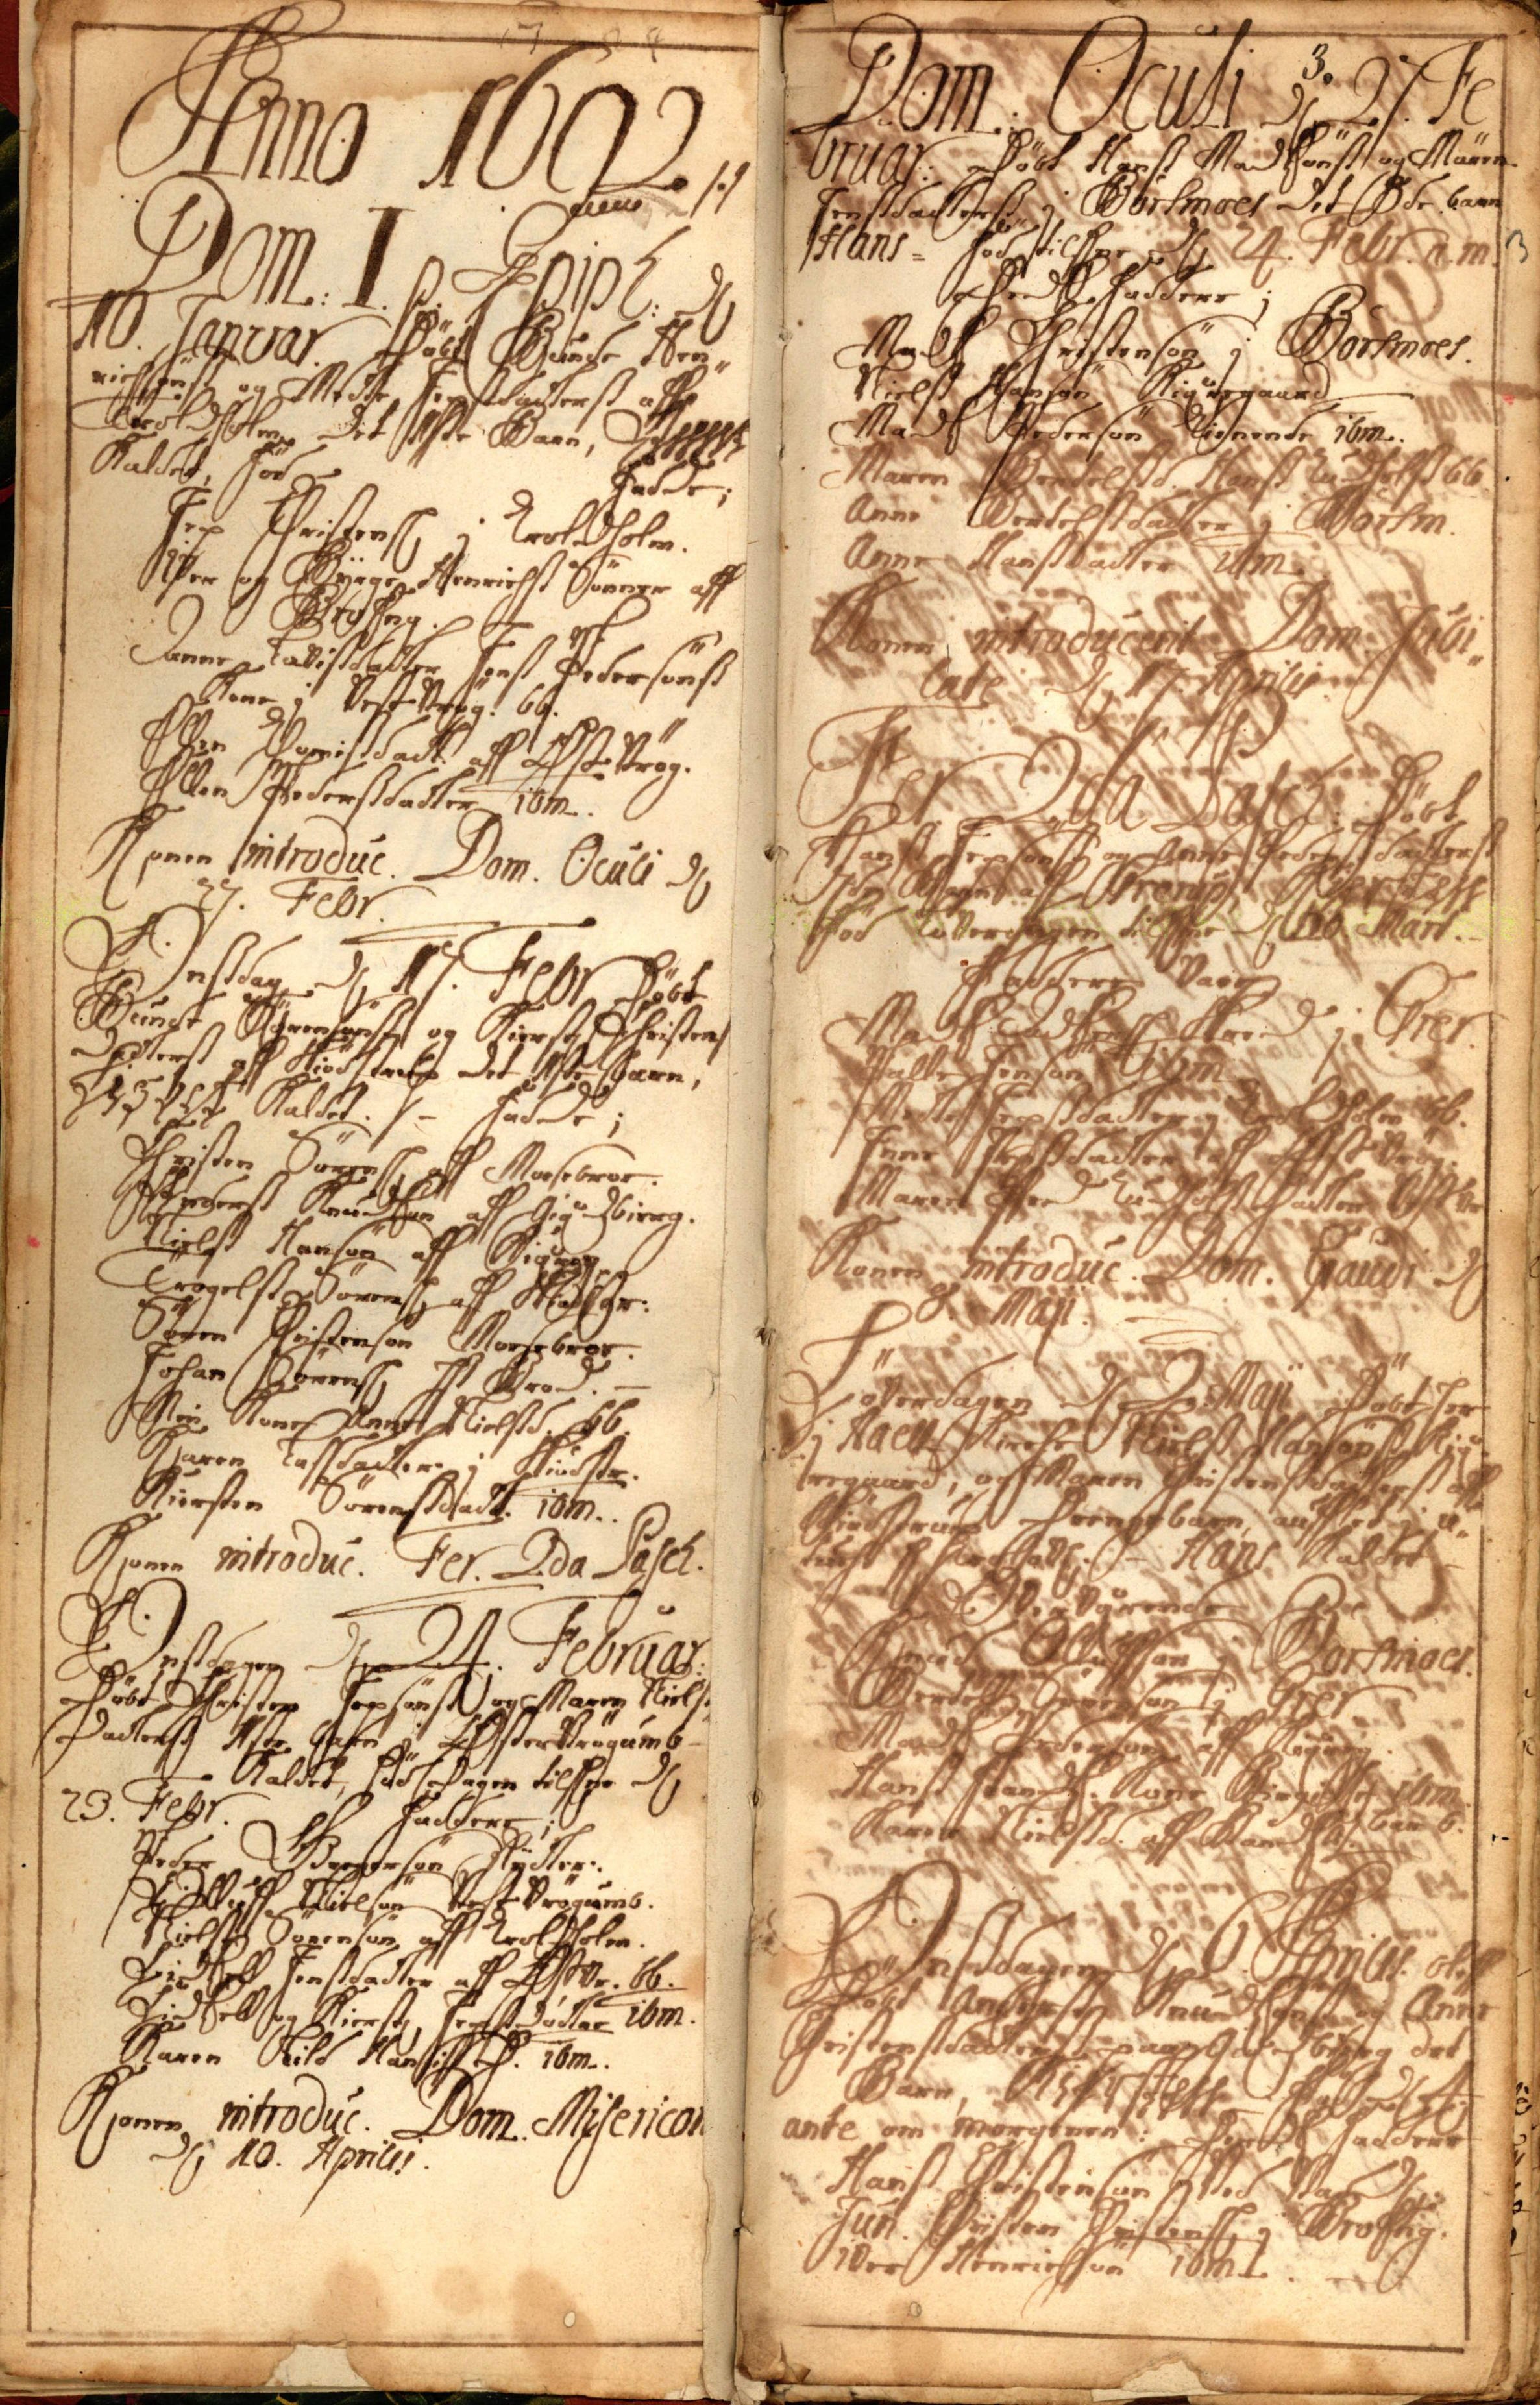Anno 1692
11
Dom:1.p.Epiph:. d
10. Januar. Døbt Bunde Hen¬
richsøn og Mette Jepsdatters aff
Troldholm det 1ste Barn, Anne
kaldet, fød
Fadder
Jep Christens j Troldholm.
Iver og Byrge Henrichs Sønner aff
BroEng.
Anne Lavisdatter Jens Pedersøns
Kone j Vester Vrøg. bb.
Ellen Thomisdatter aff Øster Vrøg.
Ellen Pedersdatter ibm.
Konen introduc. Dom. Oculi d
27. Febr.
Onsdag d 17. Febr Døbt
Bunde Sørensøn og Kirst Christens
datters aff Skødstrup det 1ste barn,
Lisbet kaldet. Fadder;
Christen Sørens aff Moesebroe.
Anders Knudsen af Giedbierg.
Niels Hansen aff Kiærgaa##,
Trogels Sørens aff Skødstr:
Søren Christensen af Moesebroe.
Johan Sørens ## Br##
Min kone Anne Nielsd. bb.
Karen Lassdatter j Skiødstr.
Kirsten Sørensdatt. ibm..
Konen introduc. Fer. 2da Pasch.
Onsdagen d 24. Februar:
Døbt Christen Jepsøns og Maren Niels¬
datters 1ste barn j Øster Vrøgumb
kaldet, fød dagen tilforn d
23. Febr.
Faddere;
Peder Gregersøn Rydter:
## Oluf Nielsøn i Vester Vrøgumb.
Niels Sørensøn aff Troldholm.
Sidsell Jensdatter aff Øst Vr. bb.
Sidsell og Kirst Jensdøtre ibm.
Karen Lild Hansis ## ibm.
Konen introduc. Dom. Misericor
d 10. Aprilis.
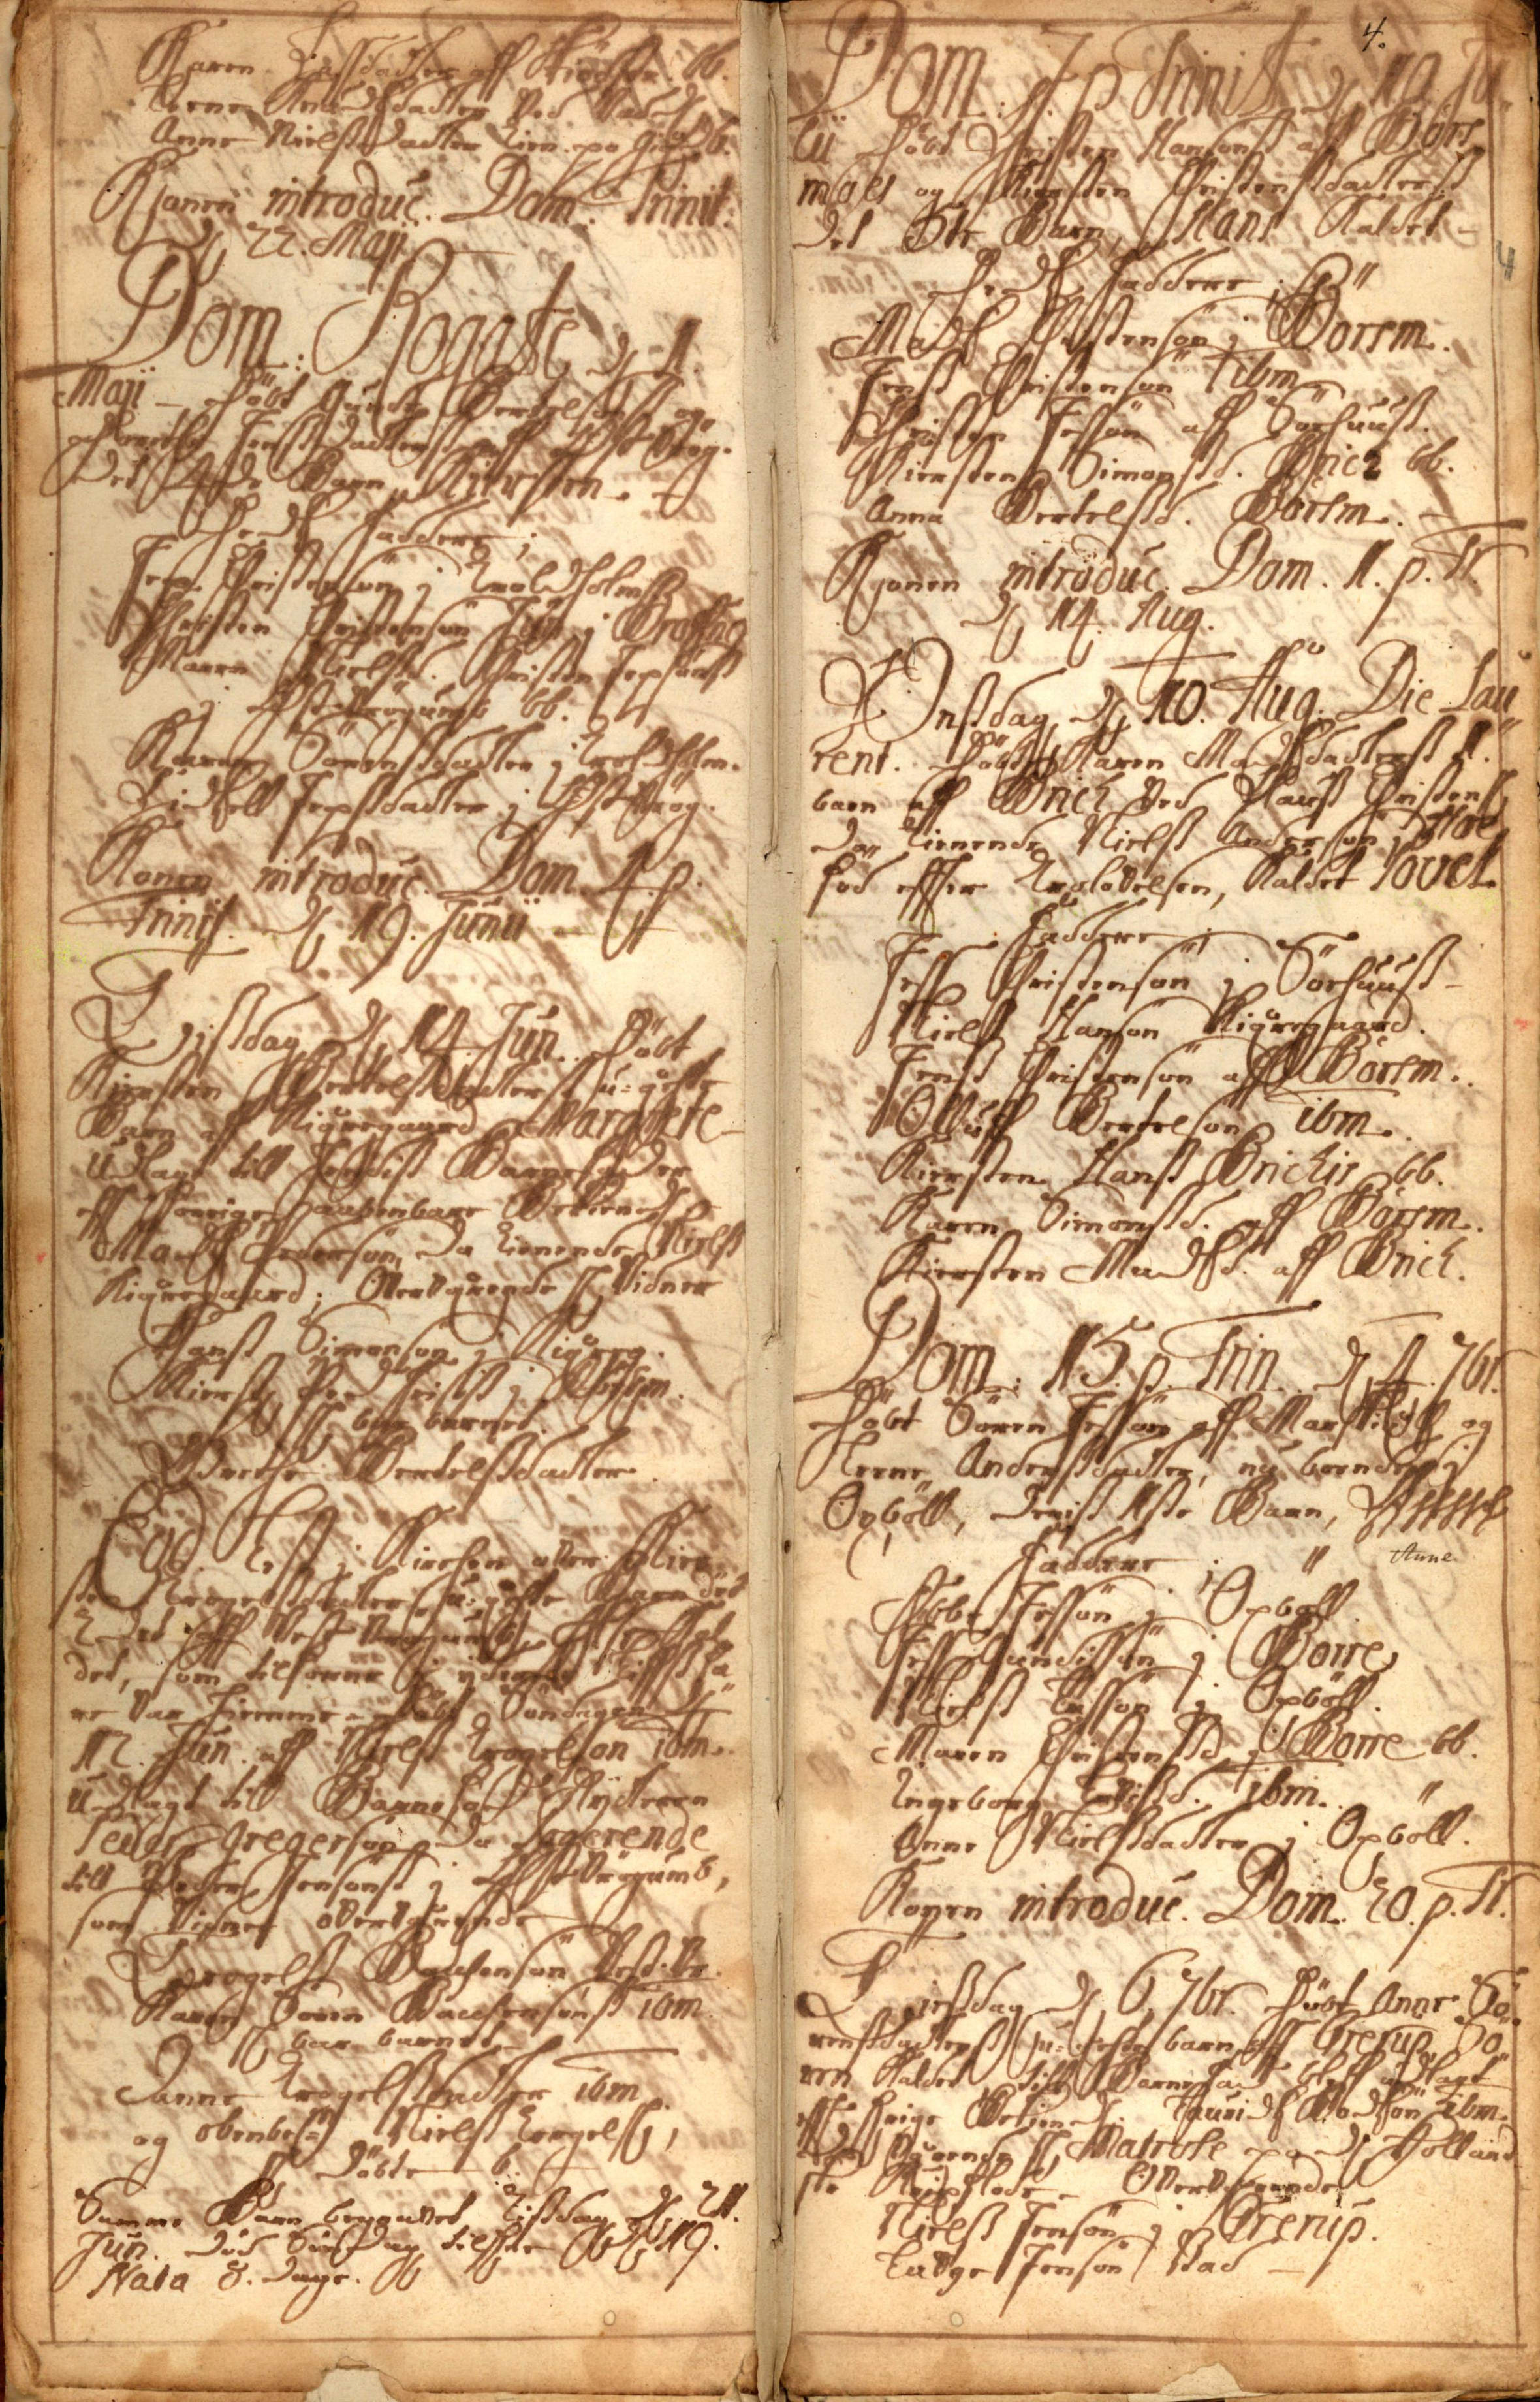4
4
Dom. 7. p.Trinit. d 10 Ju¬
lii Døbt Christen Hansens af Børs
moes og Kiersten Christensdatters
det 5te Barn, Hans kaldet
## Faddere;
Mads Christensen j Børsm
Jens Christensen ibm
Christen Jessøn aff Søehus.
Kiersten Simonsd. Brich bb.
Anne Bertelsdatter Børsm.
Konen introduc. Dom.1. p. Tr.
d 14 Aug.
Onsdag d 10. Aug. Die Lau¬
rent. Døbt Karen Madsdatters 1.
barn af Brich ved Claus Christen
sen tienende Niels Andersøn i Hoe
fød effter Trolovelsen, kaldet Povel.
Faddere,
Jess Christensen j Søehus
Niels Hansen Kjærgaard.
Jens Christensøn aff Børsm.
Oluf Bertelsøn ibm.
Kiersten Hans Brichs bb.
Karen Simonsd. aff Børsm.
Kiersten Madsd. aff Brich.
Dom.15. p. Trin. d 4. 7br.
Døbt Søren Jessøn af Marskiel og
Leene Andersdatter, nu boende j
Oxbøll, deris 1ste Barn, Anne
Anne
Faddere
Ebbe Jessen i Oxbøll.
Jess Gundesøn j Borre
Niels Lassøn j Oxbøll.
Maren Christensd j Borre bb.
Ingeborg Lavisd. ibm.
Anne Nielsdatter j Oxbøl.
Konen introduc. Dom. 20 p. Tr.
Tirsdag d 6 7br. Døbt Anne Sø¬
rensdatters u=ægte barn aff Grærup Sø¬
ren kaldet, till Barnefad blev udlagt
efft frige Bekiend Lauridtz Bodsøn ibm.
da farende s## Matro## paa d Holland
ske Skibsflode. Overværende
Niels Jensen j Grærup.
Lauge Jensen Vad